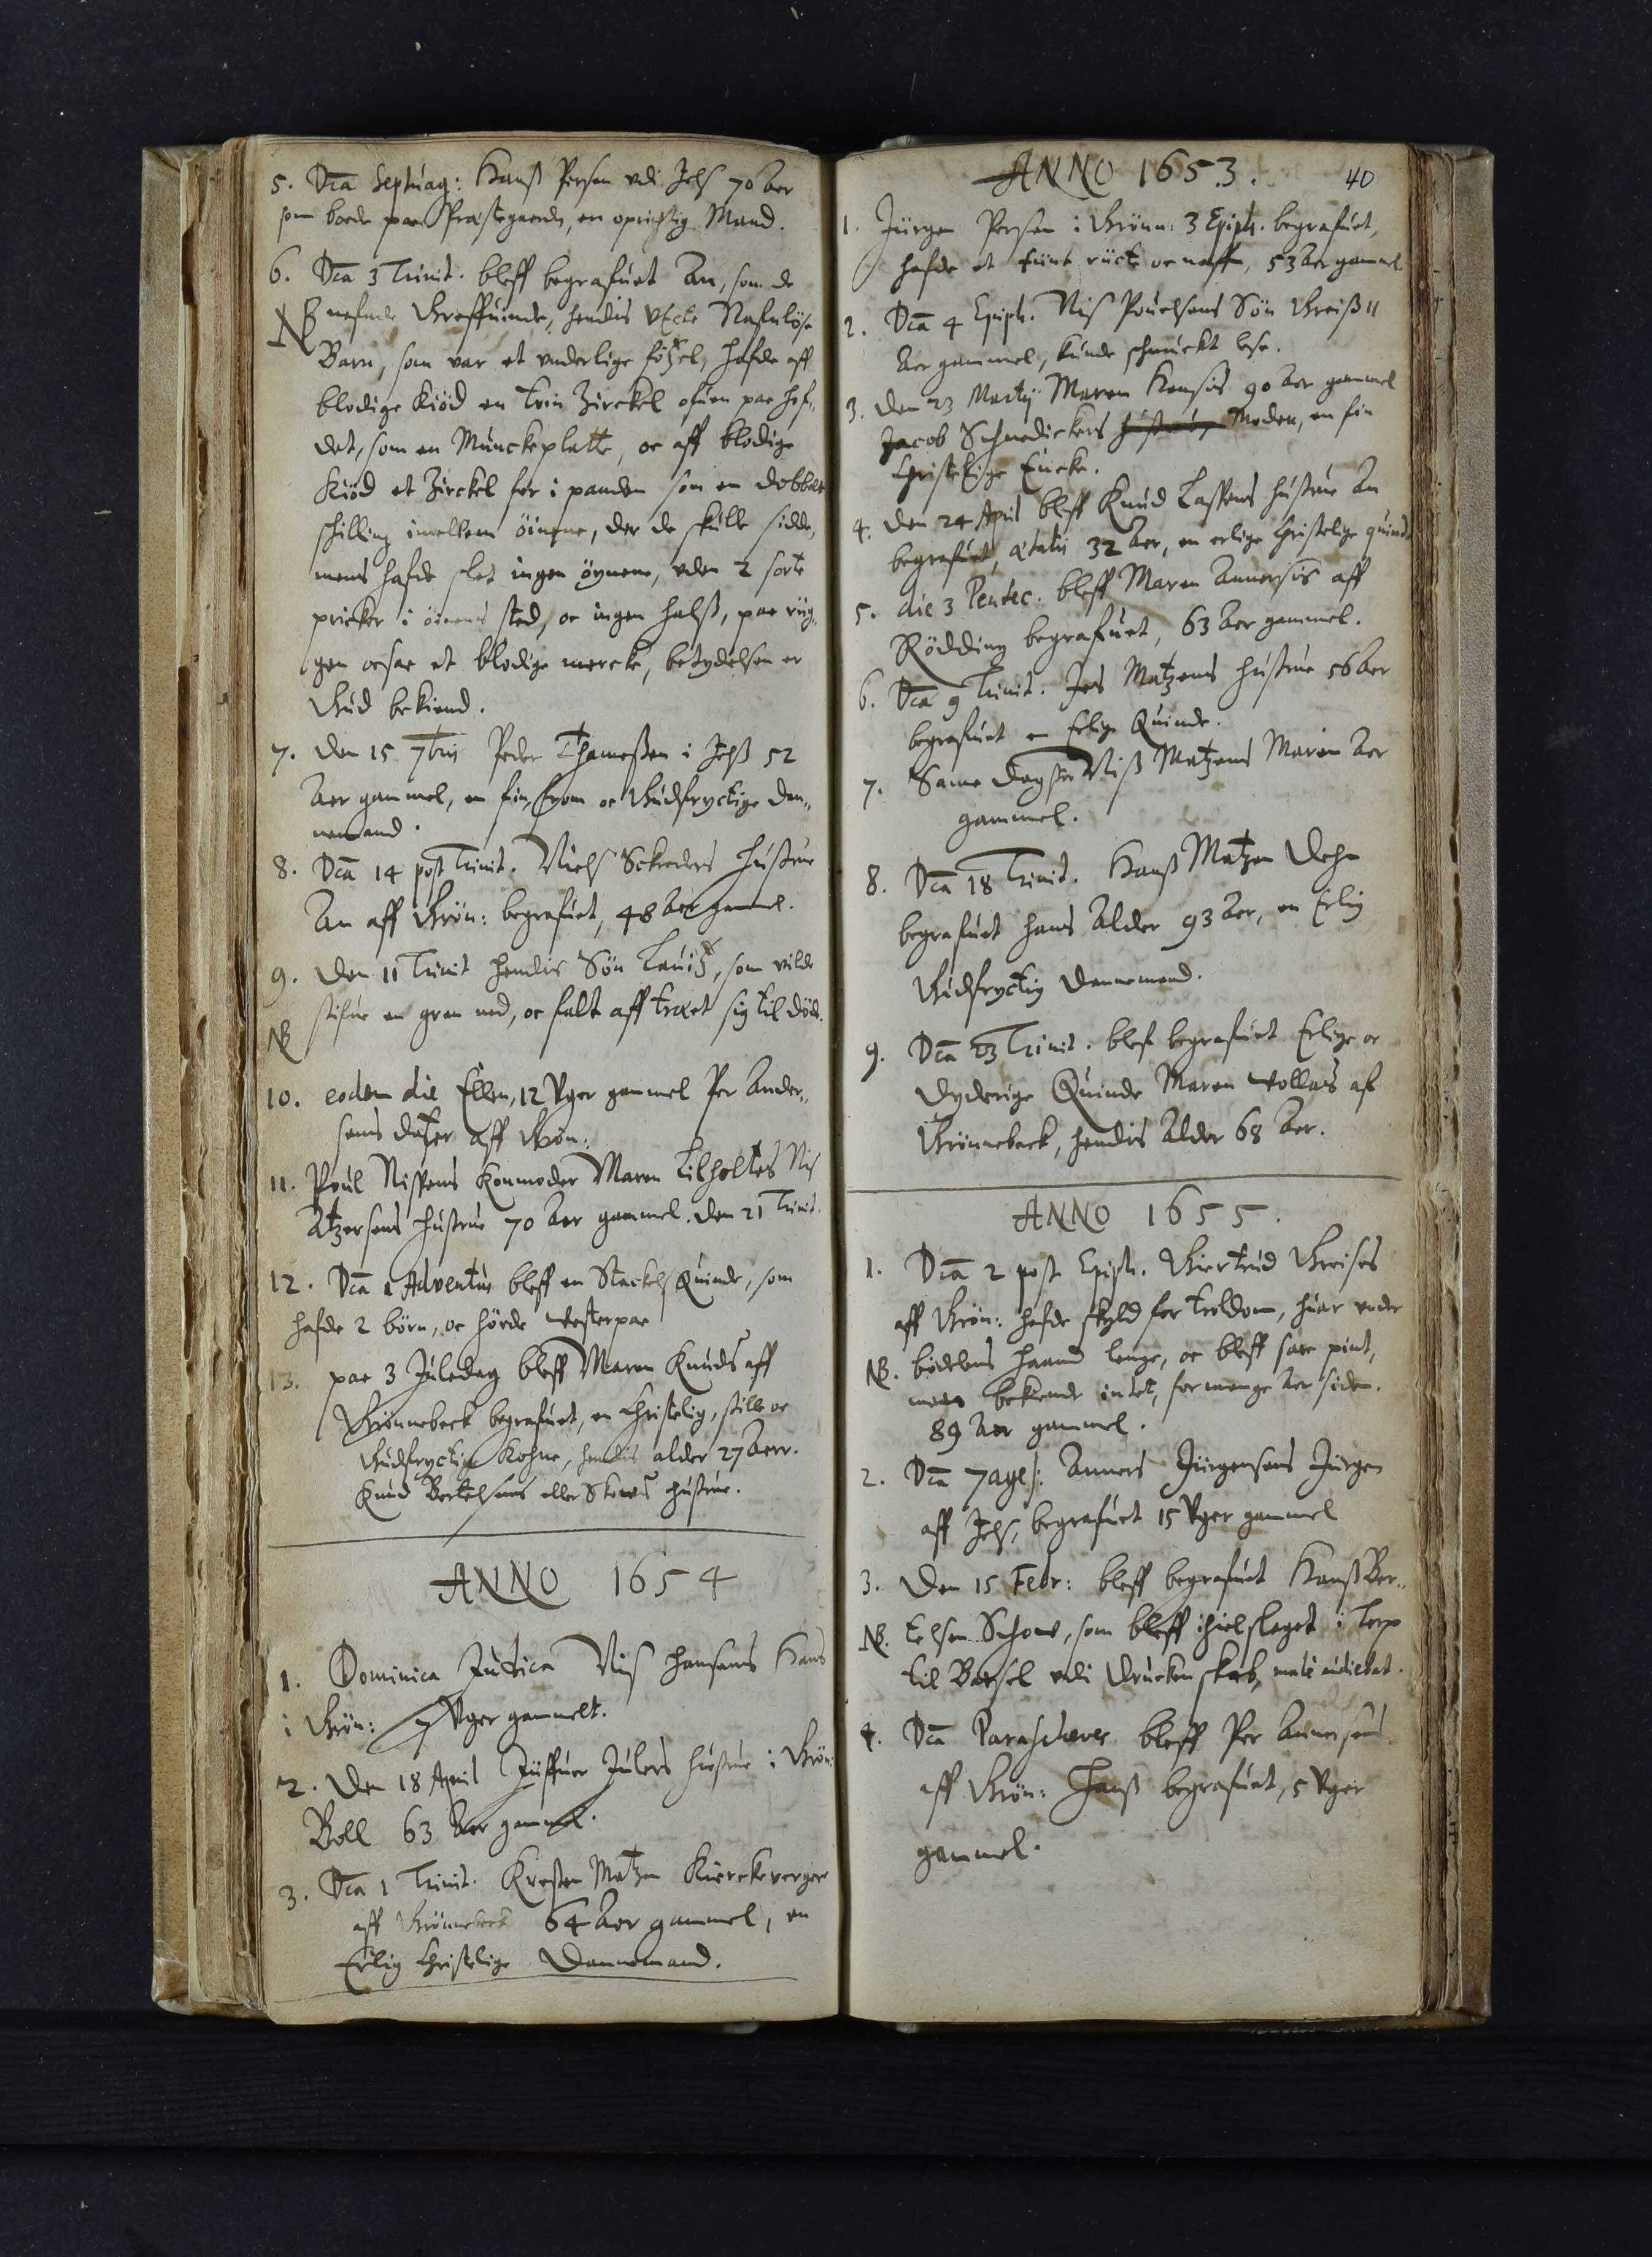5. Dca Septuag: Hans Persen udi Jels 70 Aar
som boede paa Prastegaard##, en oprichtig Mand.
6. Dca 3 Trinit. bleff begrafuet An, som de
NB nefende Graffuinde, hendis VEcte Nafnløse
Barn, som var et vnderlige føtzel, hafde aff
blodige kiød en trin Zirckel ofuen paa hof¬
det, som en Munckeplatte, oc aff blodige
kiød et Zirckel for i panden som en dobbelt
skilling imellem øinene, der de skulle sidde,
mens hafde slet ingen øynene, uden 2 sorte
pricker i øinenes sted, oc ingen halss, paa rug¬
gen ocsae et blodige mercke, betydelsen er
Gud bekiend.
7. Den 15 7tris Peder Thamessen i Jelss 52
Aar gammel, en fin, from oc Gudfryctige Dan¬
nemand.
8. Dca 14 post Trinit. Niels Sckreders hustrue
An aff Grøn: begrafuet, 48 Aar gammel.
9. Den 11 Trinit hendis Søn Lauitz, som
vilde sifue en gren ned, oc faldt aff traet sig til døde.
10. eodem die Ellen, 12 Vger gammel Per Ander¬
sens dater aff Grø:
11. Poul Nissens Konmoder Maren Lilholtes Nis
Atzersens hustrue 70 Aar gammel, den 21 Trinit.
12. Dca 1 Adventus bleff en stackels Quinde, som
hafde 2 børn, oc hørde vester pae
13. pae 3 Juledag bleff Maren Knuds aff
Grønnebeck begrafuet, en Christelig, stille oc
Gudfryctige Kohne, hendis alder 27 Aar.
Knud Bertelsens eller Sk##s hustrue.
ANNO 1654
1. Dominica Judica Nis Hansens Hans
i Grøn: 7 Vger gammelt.
2. Den 18 April Juffuer Julers hustrue i Grøn:
Boll 63 Aar gammel.
3. Dca 1 Trinit. Kresten Matzen Kierckeverge
aff Grønnebeck 64 Aar gammel, en
Erlig Christelige Dannemand.
40
ANNO 1653.
1. Jurgen Persen i Grønn: 3 Epiph. begrafuet,
hafde et fiint ructe oc naff, 53 Aar gammel.
2. Dca 4 Epiph. Nis Pouelsens Søn Greis 11
Aar gammel, kunde schmuckt lese.
3. Den 23 Martij Maren Hansis 90 Aar gammel
Jacob Schnedickers hustrue Moder, en fin
Christelige Eneke.
4. Den 24 April bleff Knud Lassens hustrue An
begrafuet, atatis 32 Aar, en erlige Christelige quinde
5. die 3 Pentec. bleff Maren Annersis aff
Rødding begrafuet, 63 Aar gammel.
6. Dca 9 Trinit. Jes Matzens hustrue 56 Aar
begrafuet en Erlige Quinde
7. Same Dag stor Niss Matzens Maria Aar
gammel.
8. Dca 18 Trinit. Hans Matzen Dehn
begrafuet hans alder 93 Aar, en Erlig
Gudfructig Dannemand.
9. Dca 23 Trinit. Blef begrafuet Erlige oc
dyderige Quinde Maren Volles af
Grønnebeck, hendis Alder 68 Aar.
ANNO 1655
1. Dca 2 post Epiph. Giertrud Greises
aff Grøn: hafde skyld for troldom, huar vnder
NB. bødlens Hannd lenge, oc bleff sare pint,
bekiende intet, for mange Aar siden.
89 Aar gammel.
2. Dca 7ages: Anners Jurgensens Jurgen
aff Jels, begrafuet 15 Vger gammel
3. Den 15 Febr: bleff begrafuet Hans Ber¬
NB.
telsen Schov, som bleff ihielslaget i Lerp
til Barsel vdi Drucken skab, male audiebat.
4. Dca Paraschever bleff Per Annersens
aff Grøn: Hans begrafuet, 5 Vger
gammel.
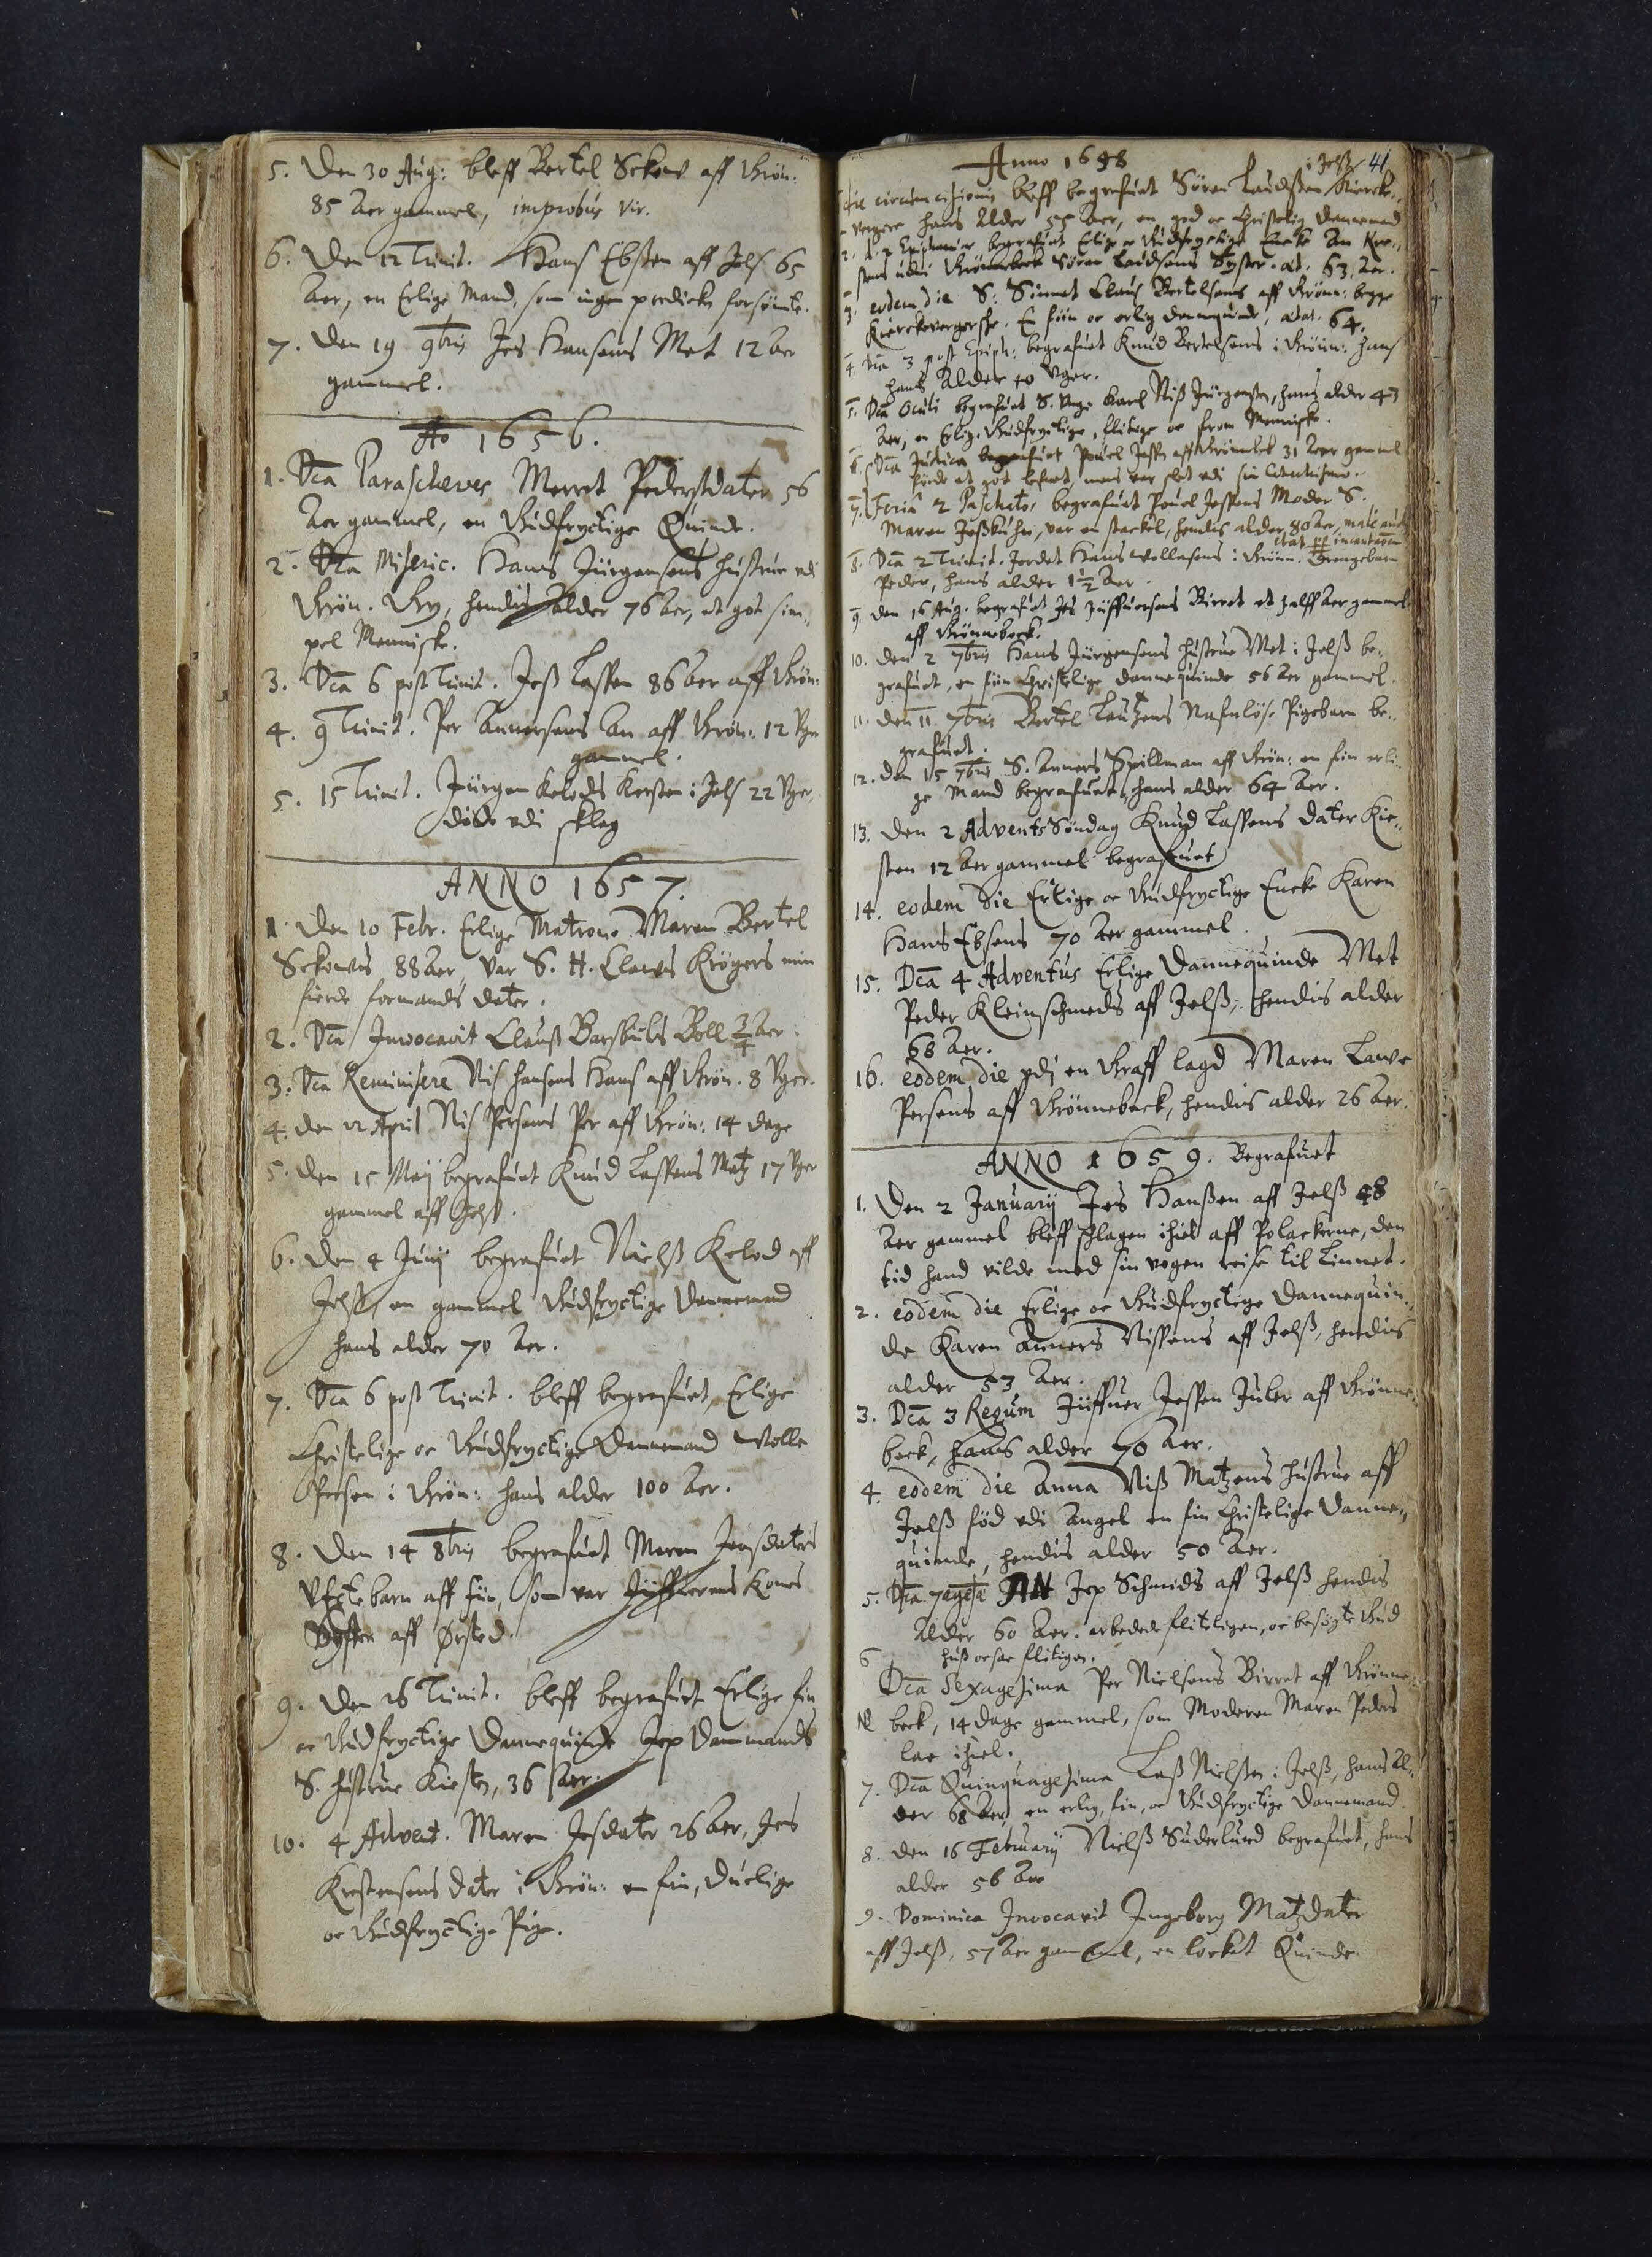5. Den 30 Aug: bleff Bertel Sckov aff Grøn:
85 Aar gammel, improbus vir 
6. Den 12 Trinit. Hans Ebssen aff Jels 65
Aar, en Erlige Mand, som ingen predicken forsømte.
7. Den 19 9tris Jes Hansens Met 12 Aar
gammel.
Ao 1656.
1. Dca Paraschever Merret Pedersdater 56
Aar gammel, en gudfryctige Quinde.
2. Dca Miseric. Hans Jurgensens Hustrue vdi
Grøn. Gry, hendes Alder 76 Aar, et got sim¬
pel Menniske.
3. Dca 6 post Trinit. Jes Lassen 86 Aar aff Grøn:
4. 9 Trinit. Per Annersens An aff Grøn: 12 Vger
gammel.
5. 15 Trinit. Jurgen Keleds Kresten i Jels 22 Vger
døde vdi
ANNO 1657.
1. Den 10 Febr. Erlige Matrone Maren Bertel
Sckovs 88 Aar, var S. H. Clavs Krøgers min
fierde formands dater.
2. Dca Invocavit Claus Barsbuls Boll ¾ Aar
3. Dca Reminisere Nis Hansens Hans aff Grøn. 8 Vger.
4. Den 12 April Nis Persens Per aff Grøn: 14 Dage
5. Den 15 Maij begrafuet Knud Lassens Matz 17 Vger
gammel aff Jels
6. Den 4 Junij begrafuet Nielss Kelod aff
Jelss, en gammel Gudfryctige Dannemand
hans alder 70 Aar.
7. Dca 6 post Trinit. Bleff begrafuet Erlige
Christelige oc Gudfryctige Dannemand Volle
Persen i Grøn: hans alder 100 Aar.
8. Den 14 8tris begrafuet Maren Jensdater
VEecte barn aff Fun, som var Juffuerens Kones
Syster aff Ørsted.
9. Den 26 Trinit. bleff begrafuet Erlige fin
oc Gudfryctige Dannequinde Jep Dannemands
S. hustrue Kirsten, 36 Aar.
10. 4 Advent. Maren Jesdater 26 Aar, Jes
Krestensens Dater i Grøn: en fin, Duelige
oc Gudfryctige Pige.
41
Anno 1698
i Jelss
circum cisionis bleff begrafuet Søren Laudssen Kierck¬
vergen hans alder 55 Aar, en god oc Christelig Dannemand
2. d. 2 Epipha## er begrafuet Erlige oc gudfryctige Encke An Kre¬
stens udi Grønnebeck Søren Laudsens Syster. at. 63 Aar.
3. eodem die S: Sinnet Claus Bertelsens aff Grøn: begge
kierckevergerske. En fiin oc erlig Dannequinde, atat. 64
4. Dca 3 post Epiph: begrafuet Knud Bertelsens i Grønn: Hans
hans Alder 40 Vger.
5. Dca Oculi begrafuet S. Vnge Karl Niss Jurgensen, hans alder 43
Aar, en Erlig, Gudfructige, flitige oc from Menniske.
6. Dca Judica begrafuet Pouel Jessen aff Grønnebeck 31 Aar gammel
førde et got lefnet, mens var slet vdi sin Catekismus.
7. Feria 2 Paschatos begrafuet Pouel Jessens Moder S.
Maren Jess Kuhn, var en stackel, hendes alder 80 Aar, male aude¬
tat## insantaum##
8. Dca 2 Trinit. Jordet Hans Vollesens i Grønn. Drengebarn
Peder, hans alder 1½ Aar
9. Den 16 Aug. Begrafuet Jes Juffuersens Birret et Halff Aar gammel
af Grønnebeck.
10. Den 2 7tris Hans Jurgensens Hustrue Met i Jelss be¬
grafuet, en fiin Christelige Dannequinde 56 Aar gammel.
11. Den 11 7tris Bertel Lautzens Nafnløse Pigebarn be¬
grafuet
12. Den 15 7tris S. Anners Spillman aff Grøn, en fin erli¬
ge Mand begrafuet, hans alder 64 Aar.
13. Den 2 Advents Søndag Knud Lassens Dater Kir¬
sten 12 Aar gammel begrafuet
14. eodem die Erlige oc Gudfryctige Encke Karen
Hans Ebsens 70 Aar gammel.
15. Dca 4 Adventus Erlige Dannequinde Met
Peder Kleinschmeds aff Jelss, hendis alder
68 Aar.
16. eodem die vdi en Graff lagd Maren Lave
Persens aff Grønnebeck, hendis alder 26 Aar.
ANNO 1659. Begrafuet
1. Den 2 Januarij Jes Hanssen aff Jelss 48
Aar gammel bleff slagen ihiel aff Polackerne, den
tid hand vilde med sin Vogen reise til Linnet.
2. eodem die Erlige oc Gudfryctige Dannequin
de Karen Anners Nissens aff Jelss, hendis
alder 53 Aar.
3. Dca 3 Regum Juffuer Jessen Juler aff Grønne¬
beck, hans alder 70 Aar.
4. eodem die Anna Niss Matzens Hustrue aff
Jelss fød vdi Angel en fin Christelige Danne¬
quinde, hendis alder 50 Aar.
5. Dca 7agesa AN Jep Schmids aff Jelss hendis
Alder 60 Aar, arbedede fliteligen, oc besøgte Gud
huss ocsae flitigen.
6
Dca Sexagesima Per Nielsens Birret aff Grønne¬
NB beck, 14 Dage gammel, som Moderen Maren Peders
lae ihiel.
7. Dca Quinguagesima Lass Nielssen i Jelss, hans Al¬
der 68 Aar, en erlig, fin, oc Gudfryctige Dannemand.
8. Den 16 Februarij Nielss Suderlund begrafuet, hans
alder 56 Aar
9. Dominica Invocavit Ingeborg Matzdater
aff Jelss, 57 Aar gammel, en locket Quinde